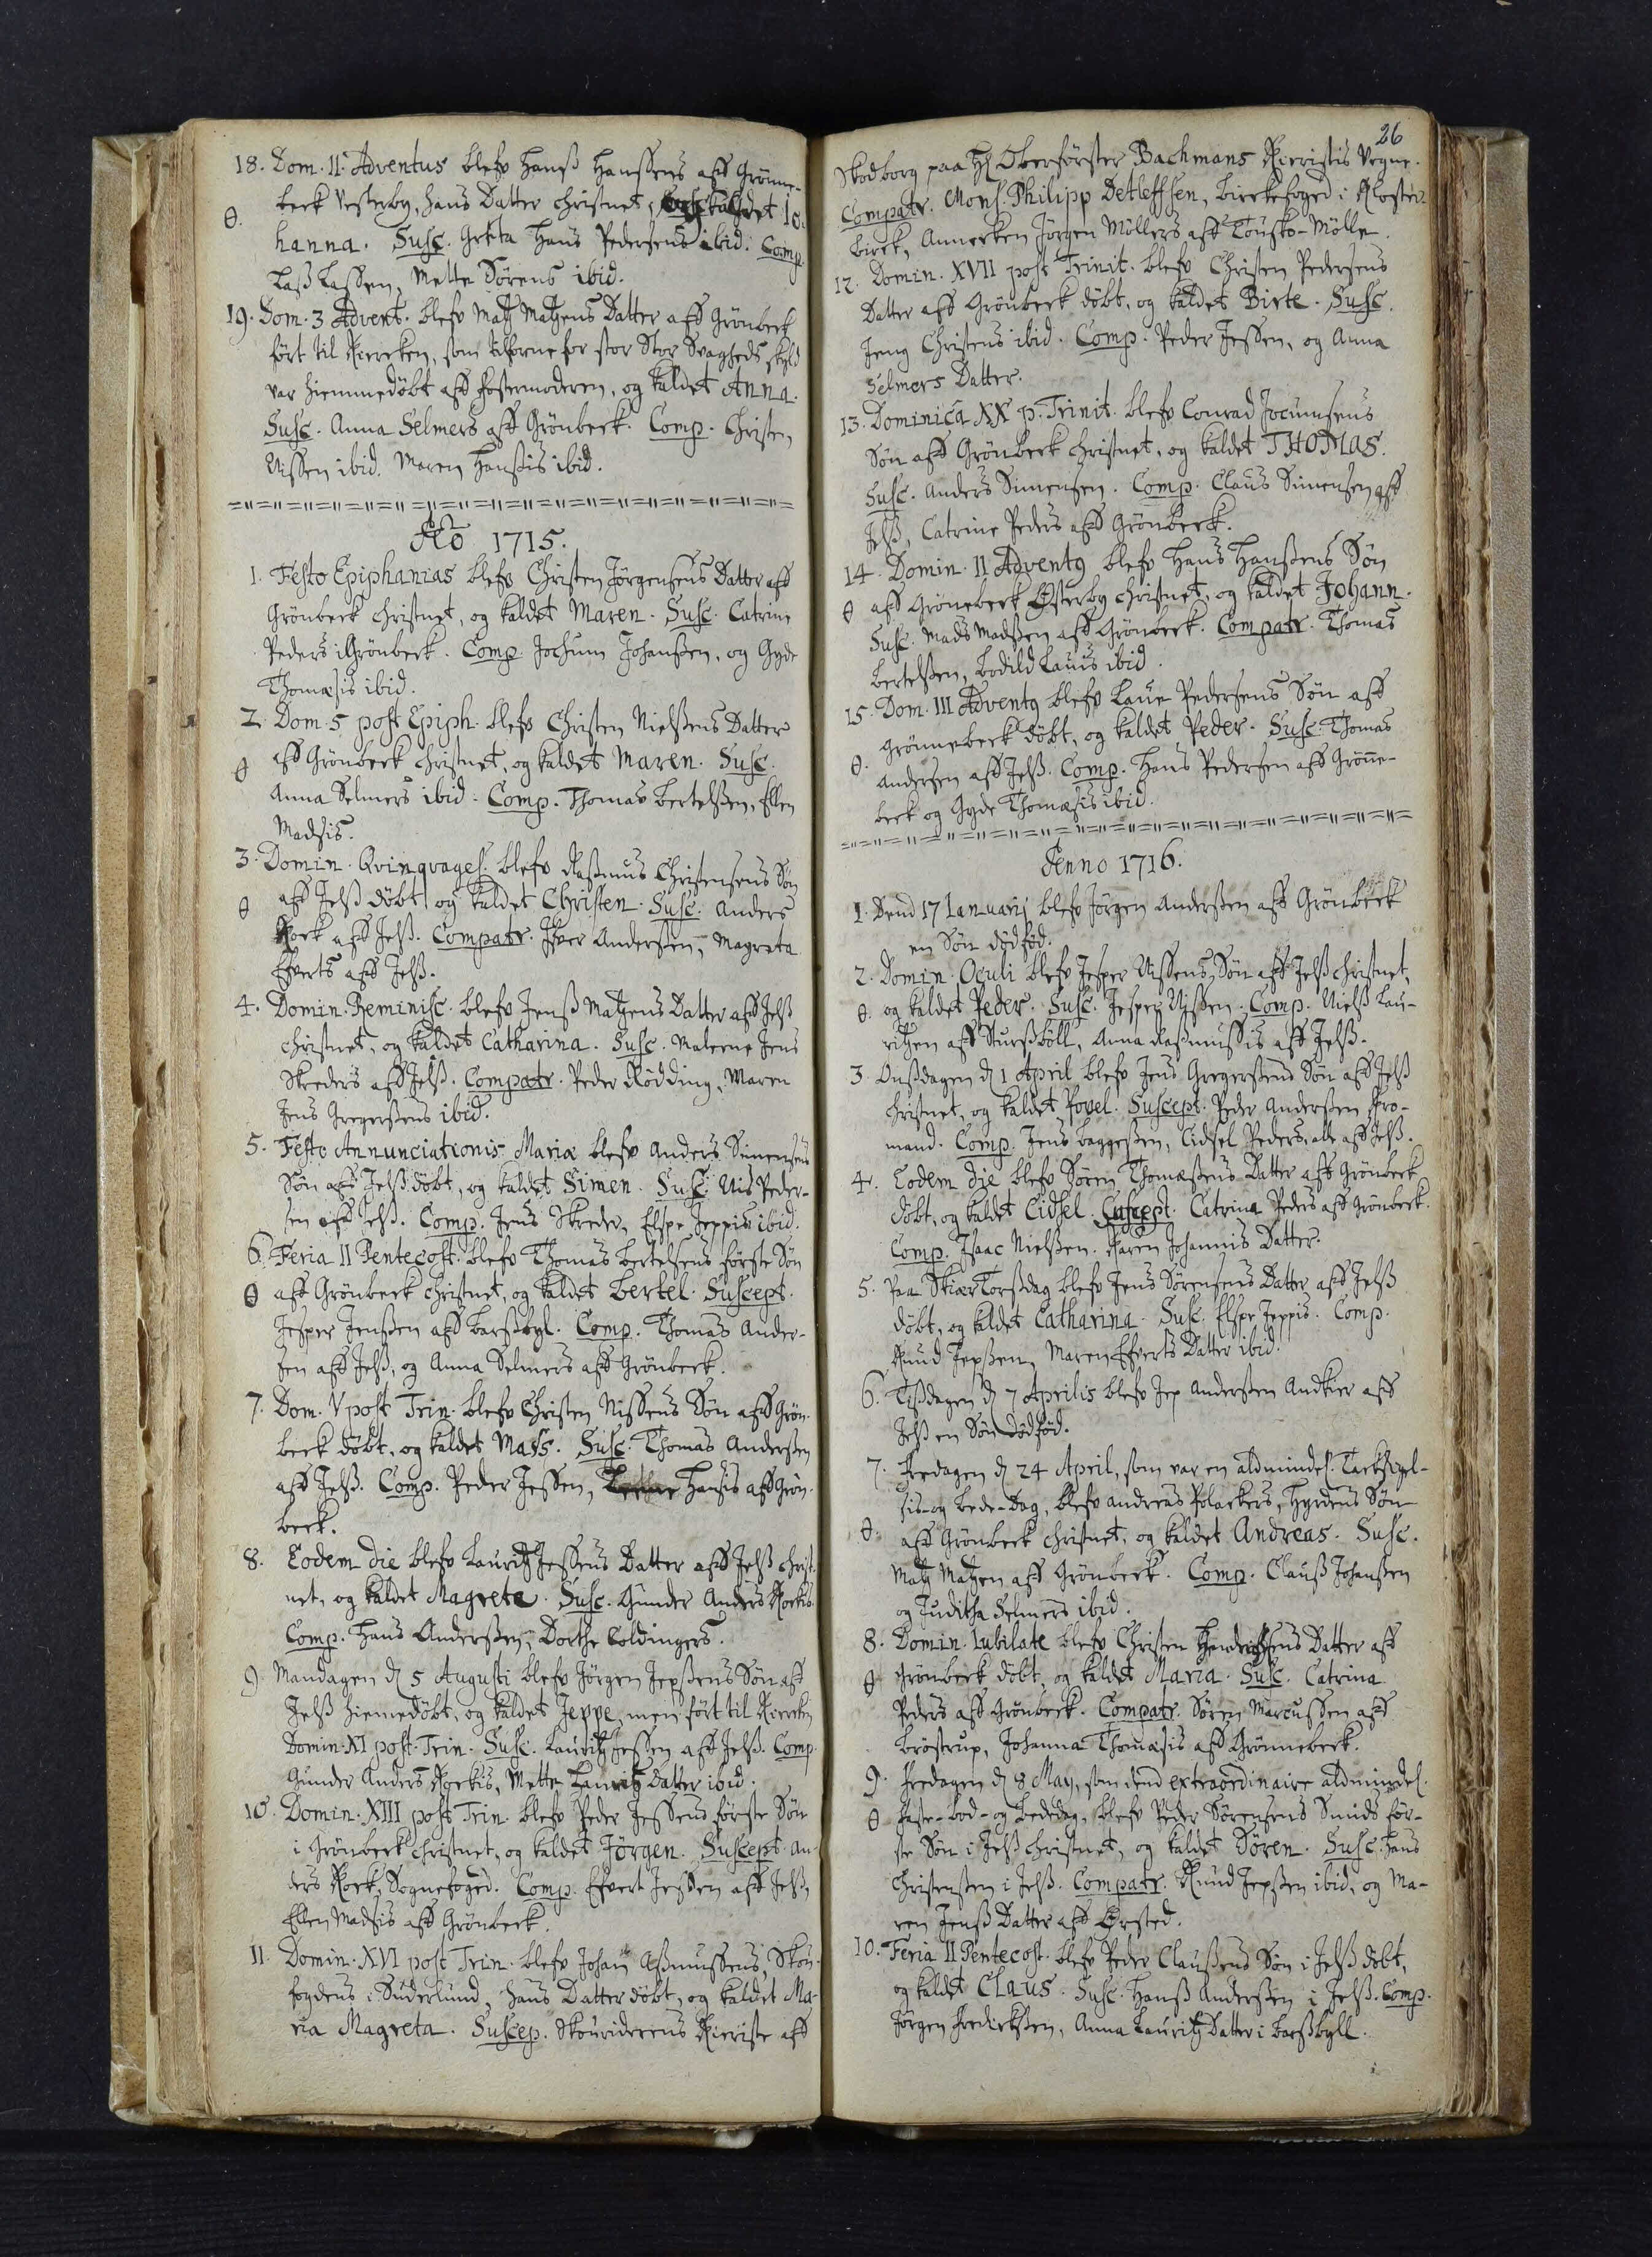18. Dom. II Adventus blefv Hans Hanssens aff Grønne¬
beck Vesterby, hans Datter christnet, og kaldet Io¬
hanna. Susc. Greta## Hans Pedersens ibid. Comp.
Lass Lassen, Mette Sørens ibid.
19. Dom. 3 Advent blefv Matz Matzens Datter aff Grønbeck
ført til Kiercken, som tilforne for stor Svagheds skyld
var hiemmedøbt aff Fostermoderen, og kaldet Anna.
Susc. Anna Selmers aff Grønbeck. Comp. Christen
Nissen ibid. Maren Hansis ibid.
Ao 1715
1. Festo Epiphanias blefv Christen Jørgensens Datter aff
Grønbeck christnet, og kaldet Maren. Susc. Catrine
Peders i Grønbeck. Comp. Jochum Johanssen, og Gyde
Thomasis ibid.
2. Dom. 5 post Epiph. blefv Christen Nielssens Datter
aff Grønbeck christnet, og kaldet Maren. Susc.
Anna Selmers ibid. Comp. Thomas Bertelsen, Ellen
Madsis.
3. Domin. Qvinqvages. blefv Rassmus Christensens Søn
aff Jelss døbt og kaldet Christen. Susc. Anders
Kock aff Jelss. Compatr. Ifver Andersen, Magrete
Efverts aff Jelss.
4. Domin. Reminisc. blef Jenss Matzens Datter aff Jelss
christnet, og kaldet Catharina. Susc. Maleene Jens
Skreders aff Jelss. Compatr. Peder Rødding, Maren
Jens Gregerssens ibid.
5. Festo Annunciationis Maria blefv Anders Simensens
Søn aff Jelss døbt, og kaldet Simen. Susc. Nis Peder¬
sen aff Jelss. Comp. Jens Skreder, Elspe Jeppis ibid.
6. Feria II Pentecost blefv Thomas Bertelsens første Søn
aff Grønbeck christnet, og kaldet Bertel. Suscept.
Jesper Jenssen aff Barssbyl. Comp. Thomas Ander¬
sen aff Jelss, og Anna Selmers aff Grønbeck.
7. Dom. V post Trin. blefv Christen Nissens Søn aff Grøn¬
beck døbt, og kaldet Mads. Susc. Thomas Andersen
aff Jelss. Comp. Peder Jessen, Leene Hansis aff Grøn¬
beck.
8. Eodem die blefv Lauritz Jessens Datter aff Jelss christ¬
net, og kaldet Magreta. Susc. Gunder Anders Kockis.
Comp. Hans Anderssen, Dorthe Coldingers.
9. Mandagen d 5 Augusti blefv Jørgen Jepssens Søn aff
Jelss hiemedøbt, og kaldet Jeppe, men ført til Kiercken
Domin. XI post Trin. Susc. Lauritz Jessen aff Jelss. Comp.
Gunder Anders Kockis, Mette Lauritz Datter ibid
10. Domin. XIII post Trin blefv Peder Jessens første Søn
i Grønbeck christnet, og kaldet Jørgen. Suscept. An¬
ders Kock, Sognefoged. Comp. Efvert Jessen aff Jelss,
Ellen Madsis aff Grønbeck
11. Domin. XVI post Trin. blefv Johan Assmussens, Skou¬
fogdens i Suderlund, hans Datter døbt, og kaldet Ma¬
ria Magreta. Suscep. Skouriderens Kieriste aff
26
Skodborg paa Hl Oberførster Bachmans Kieristis Vegne.
Compatr. Mons. Philipp Detleffsen, Birckefoged i Kloster¬
birck, Annecken Jørgen Møllers aff Tousko-Mølle.
12. Domin. XVII post Trinit. blefv Christen Pedersens
Datter aff Grønbeck døbt, og kaldet Birte. Susc.
Jeng Christens ibid. Comp. Peder Jessen, og Anna
Selmers Datter.
13. Dominica XX p. Trinit. blefv Conrad Jocumsens
Søn aff Grønbeck christnet, og kaldet THOMAS.
Susc. Anders Simensen. Comp. Claus Simensen aff
Jelss, Catrine Peders aff Grønbeck.
14. Domin. II Adventq blefv Hans Hansens Søn
aff Grønebeck Østerby christnet, og kaldet Johann.
Susc. Mads Madssen aff Grønbeck. Compatr. Thomas
Bertelsen, Bodild Lauis ibid
15. Dom III Adventq blefv Laue Pedersens Søn aff
Grønnebeck døbt, og kaldet Peder. Susc. Thomas
Andersen aff Jelss. Comp. Hans Pedersen aff Grønne¬
beck og Gyde Thomæsis ibid.
Anno 1716.
1. Dend 17 Ianuarij blefv Jørgen Anderssen aff Grønbeck
en Søn dødfød.
2. Domin. Oculi blefv Jesper Nissens Søn aff Jelss christnet,
og kaldet Peder . Susc. Jesper Nissen. Comp. Nielss Lau¬
ritzen aff Sturssbøll, Anna Rassmussis aff Jelss.
3. Onsdagen d 1 April blefv Jens Gregersens Søn aff Jelss
christnet, og kaldet Povel. Suscept. Peder Andersen Kro¬
mand. Comp. Jens Baggessen, Cidsel Peders, alle aff Jelss.
4. Eodem die blefv Søren Thomæsens Datter aff Grønbeck
døbt, og kaldet Cidsel. Suscept. Catrina Peders aff Grønbeck
Comp. Isaac Nielssen. Karen Johannis Datter.
5. Paa Skiar Torsdag blefv Jens Sørensens Datter aff Jelss
døbt, og kaldet Catharina. Susc. Elspe Jeppis. Comp.
Knud Jepssen, Maren Efverts Datter ibid.
6. Tisdagen d 7 Aprilis blefv Jep Anderssen Andkier aff
Jelss en Søn dødfød.
7. Fredagen d 24 April, som var en aldmindel. Tacksigel¬
sis- og Bede-Dag, blefv Andreas Polackers, Hyrdens Søn
aff Grønbeck christnet, og kaldet Andreas . Susc.
Matz Matzen aff Grønbeck. Comp. Clauss Johansen
og Juditha Selmers ibid.
8. Domin. Iubilate blefv Christen Hendrichsens Datter aff
Grønbeck døbt, og kaldet Maria . Susc. Catrina
Peders aff Grønbeck. Compatr. Søren Marcussen aff
Brøstrup, Johanna Thomasis aff Grønnebeck.
9. Fredagen d 8 May, som dend extraordinaire aldmindel.
faste- bod- og bededag, blefv Peder Sørensens Smids før¬
ste Søn i Jelss christnet, og kaldet Søren . Susc. Hans
Christensen i Jelss. Compatr. Knud Jepssen ibid, og Ma¬
ren Jenss Datter aff Ørsted.
10. Feria II Pentecost. blefv Peder Claussens Søn i Jelss døbt,
og kaldet Claus. Susc. Hans Anderssen i Jelss. Comp.
Jørgen Fredicksen, Anna Lauritz Datter i Barssbyll